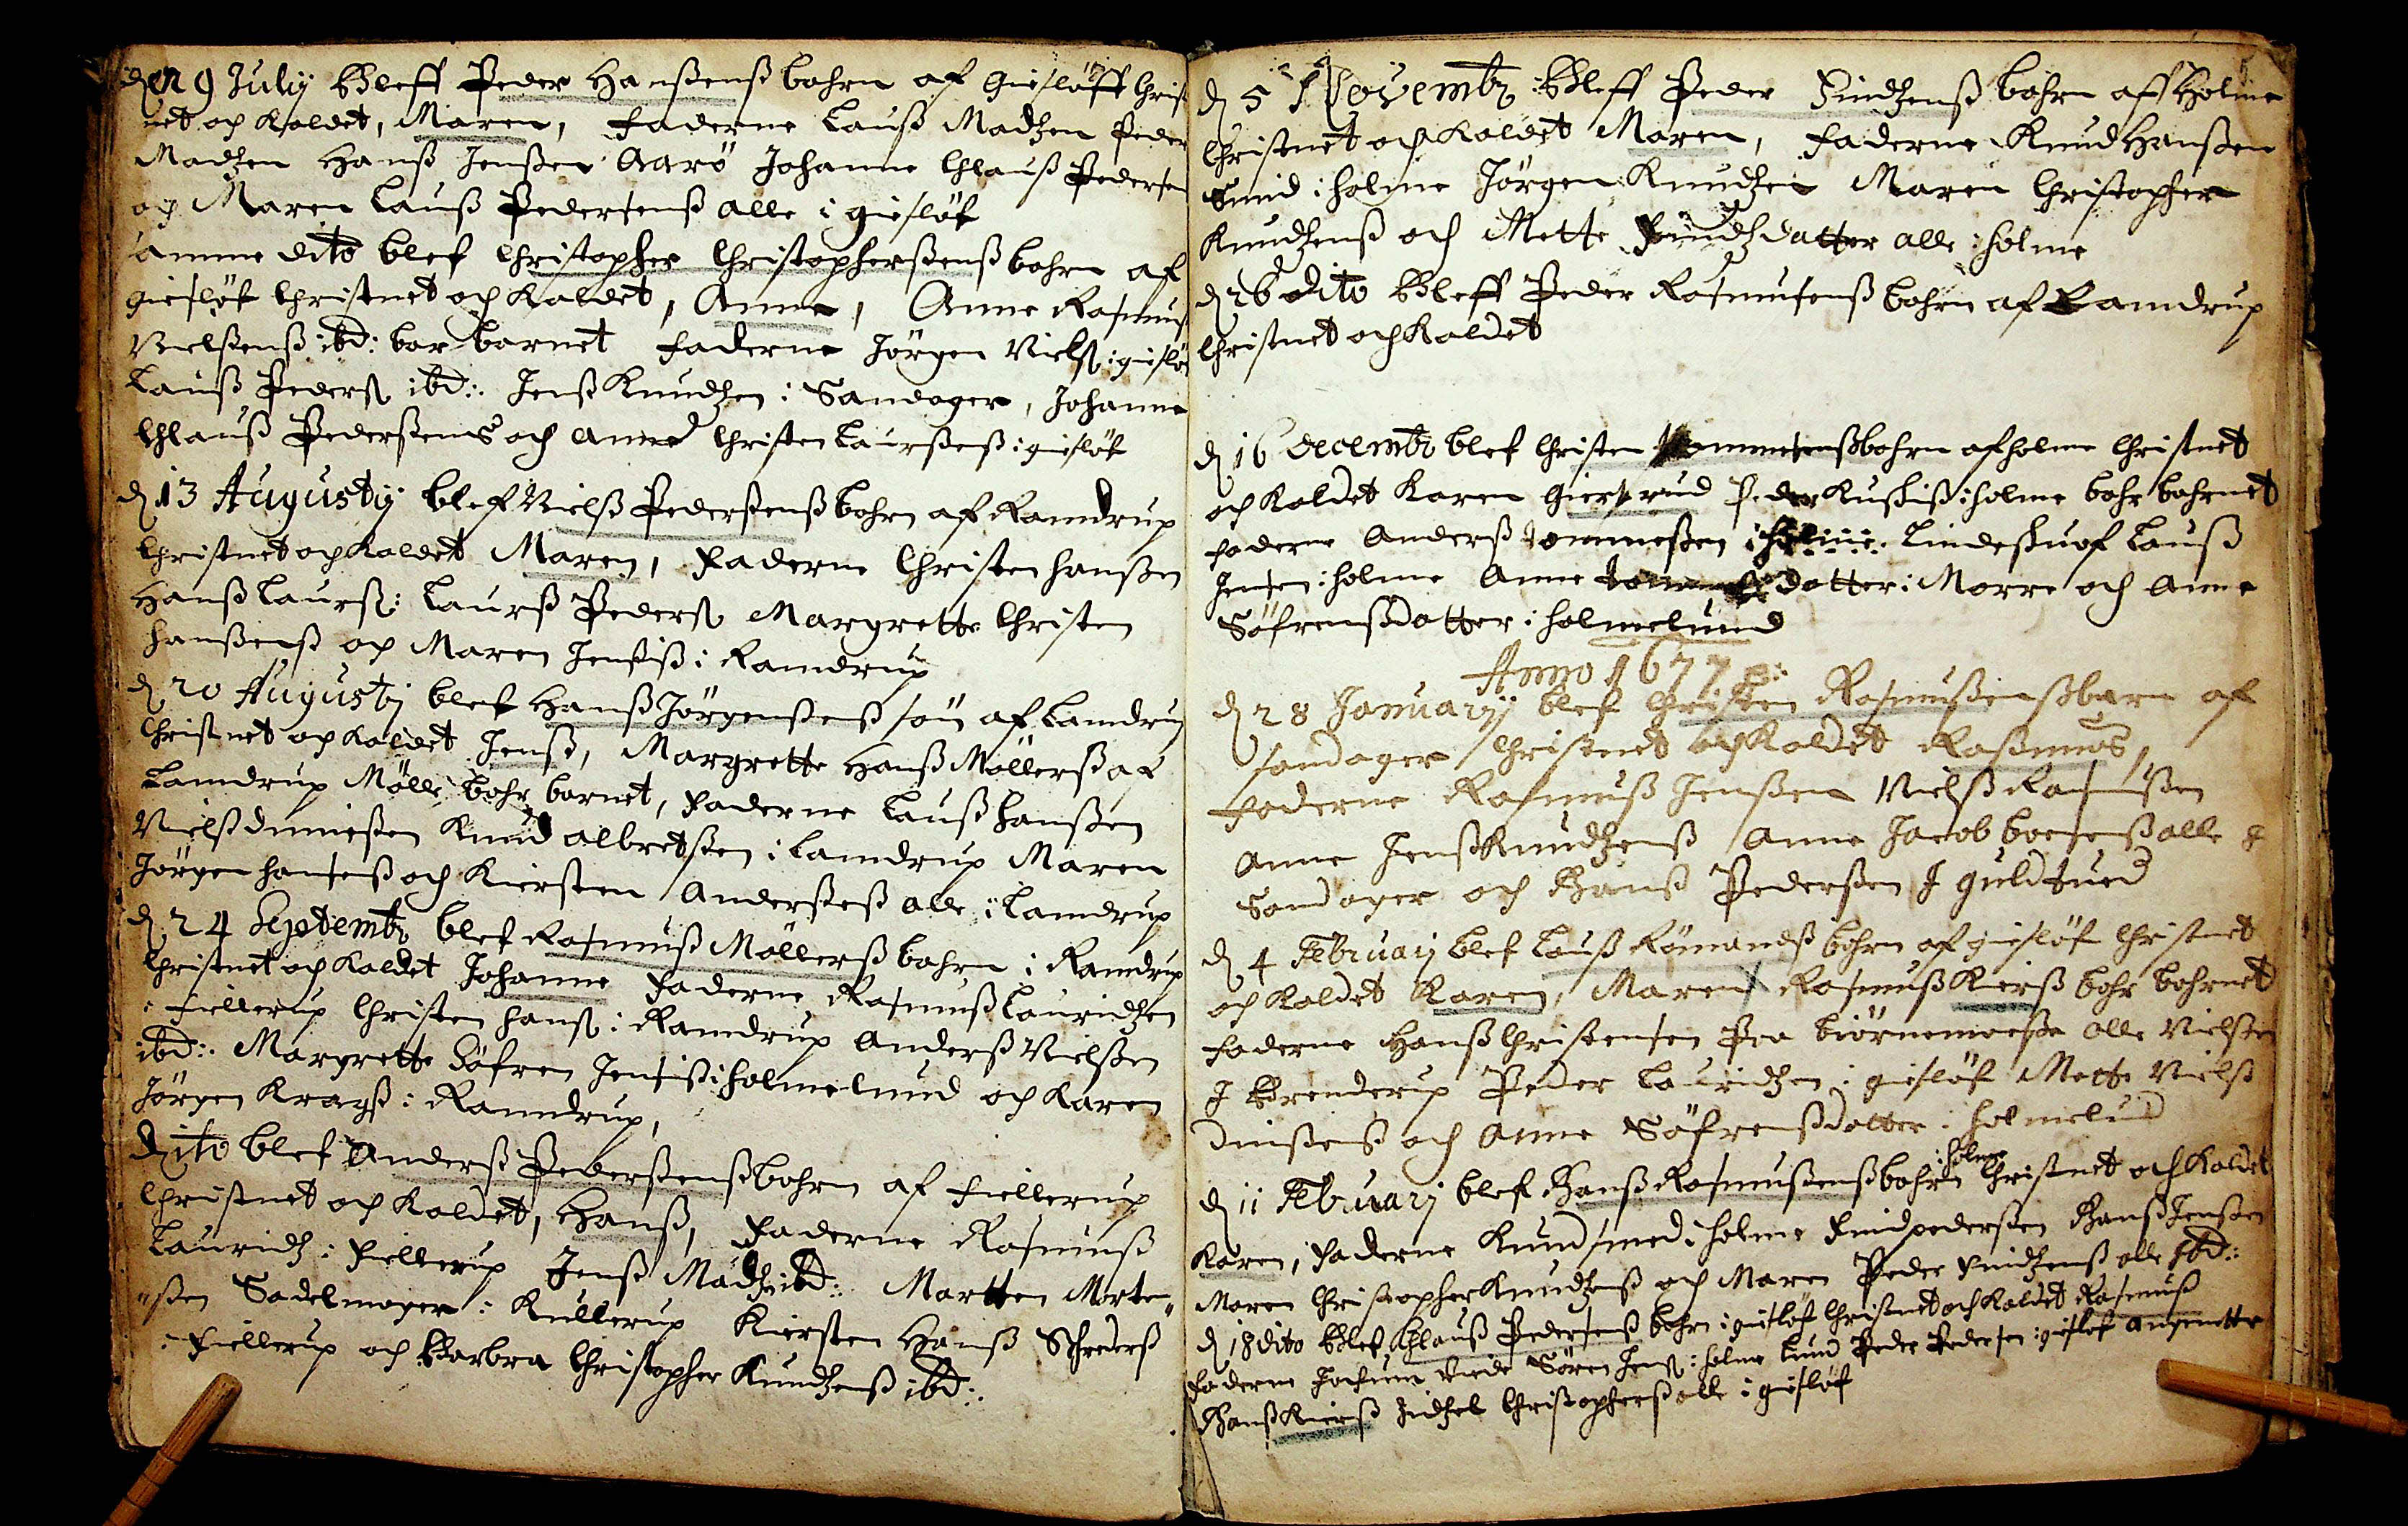den 9 Julij Bleff Peder Hanssenss Bahrn af Giesløff Christ
net och kaldet, Maren, Faderne Lauss Madtzen Peder
Madtzen Hanss Jenssen Aarø Johanne Chlaus Pedersen
och Maren Lauss Pedersensa alle i giesløf
samme dito blef Christopher Christopherssenss bahrn af
giesløf christnet och kaldet, Anne, Anne Rasmus 
Nielssenss ibd: bar barnet Faderne Jørgen Niels i gieslø
Lauss Peders ibd:. Jens Knudtzen i Sandager, Johanne
Chlaus Pederssens och Anne Christen Laurssenss i giesløf
d 13 Augustij bleff Nielss Pederssenss bahrn af Ramdrup
Christnet och kaldet Maren, Faderne Christen Hanssen
Hanss Laurss: Laurs Pederss Margrette Christen
Henssenss och Maren Jenssiss i Ramdrup
d 20 Augustj blef Hanss Jørgenssenss søn af Lamdrup
christenet och kaldet Jenss, Margrette Hanss Møllerss af
Lamdrup Mølle bahr barnet, faderene Lauss Hanssen
Niels D##iessen Knud albretssen i Lamdrup Maren
Jørgen Hansenss och Kiersten Andersens alle i Lardrup
d 24 Septembr blef Rasmuss Møllerss bahrn i Rarndrup
christnet och kaldet Johanne Faderne Rasmuss Lauridtzen
i Fiellerup Christen Hanss i Ramdrup Anderss Nielssen
ibd:. Margrette Søfren Jensiss i Holmelund och Karen
Jørgen Kragss i Ramdrup,
dito blef Anderss Pederssenss Bahrn af Fiellerup
christnet och kaldet, Hanss, Faderene Rasmuss
Lauridtz i Fiellerup Jenss Madtzen ibd:. Morten Morten¬
ssen Sadelmoger i Kullerup Kiersten Hanss Schrederss
i Fiellerup och Barbra Christopher Knudtzens ibd:.
5.
d 5 7 ##embr Bleff Peder ##Findtzenss bahrn aff Holme
christnet och Kaldet Maren, Faderne Knud Hanssen
Smid i Holme Jørgen Knudtzes Maren Christopher
Knudtzens och Mette ##Findtzdatter alle i Holme
d 26 dito Bleff Peder Rasmusenss bahrn af Ramdrup
christnet och kaldet
d 16 decembr blef Christen ##tommesenss bahrn af holme christnet
och kaldet Karen Giertrud Peder Kuskis## i holme bahr bahrnet
Fadere Anderss tommesen i Holme Lindeskuof Lauss
Jensen i holme Anne tommesdatter i Morre och Anne
Søfrenssdatter i Holmelund
Anno 1677
d 28 Januarij blef Christien Rasmussenss barn af
sandager christned och kaldet Rassmus,
faderne Rasmuss Jenssen Nielss Rasmussen
Anne Jens Knudtzenss Anne Jacob boesess alle I
Sandager och Hanss Pederssen I Guldtue##
d 4 Februarj blef Lauss Rønnendss## bahrn af giesløf christnet
och kaldet Karen, Maren Rasmuss Kierss bahr bahrnet
Faderne Hanss Christensen P## biørnemoste## Olle Nielssen
I Brenderup, Peder Lauridtzen i giesløf Mette Nielss
dinssenss## och Anne Søfrenssdatter i Holmelund
i Holme
d 11 Februuarj blef Hanss Rasmussenss bahrn christnet och kaldet
Karen, Faderne Knud smed i Holme Find pederssen Hanss Jenssen
Maren Christopher Knudtzens och Maren Peder Findtzens alle ibd.:
D 18 dito blef Chlaus Pedersenss bahrn i giesløf christenet och kaldet Rasmuss
Faderne Jochum viede## Søren Jenss i holme Lund Peder Pedersen i giesløf angnette
Hans Kierss Zidtzel Christopherss alle i giesløf
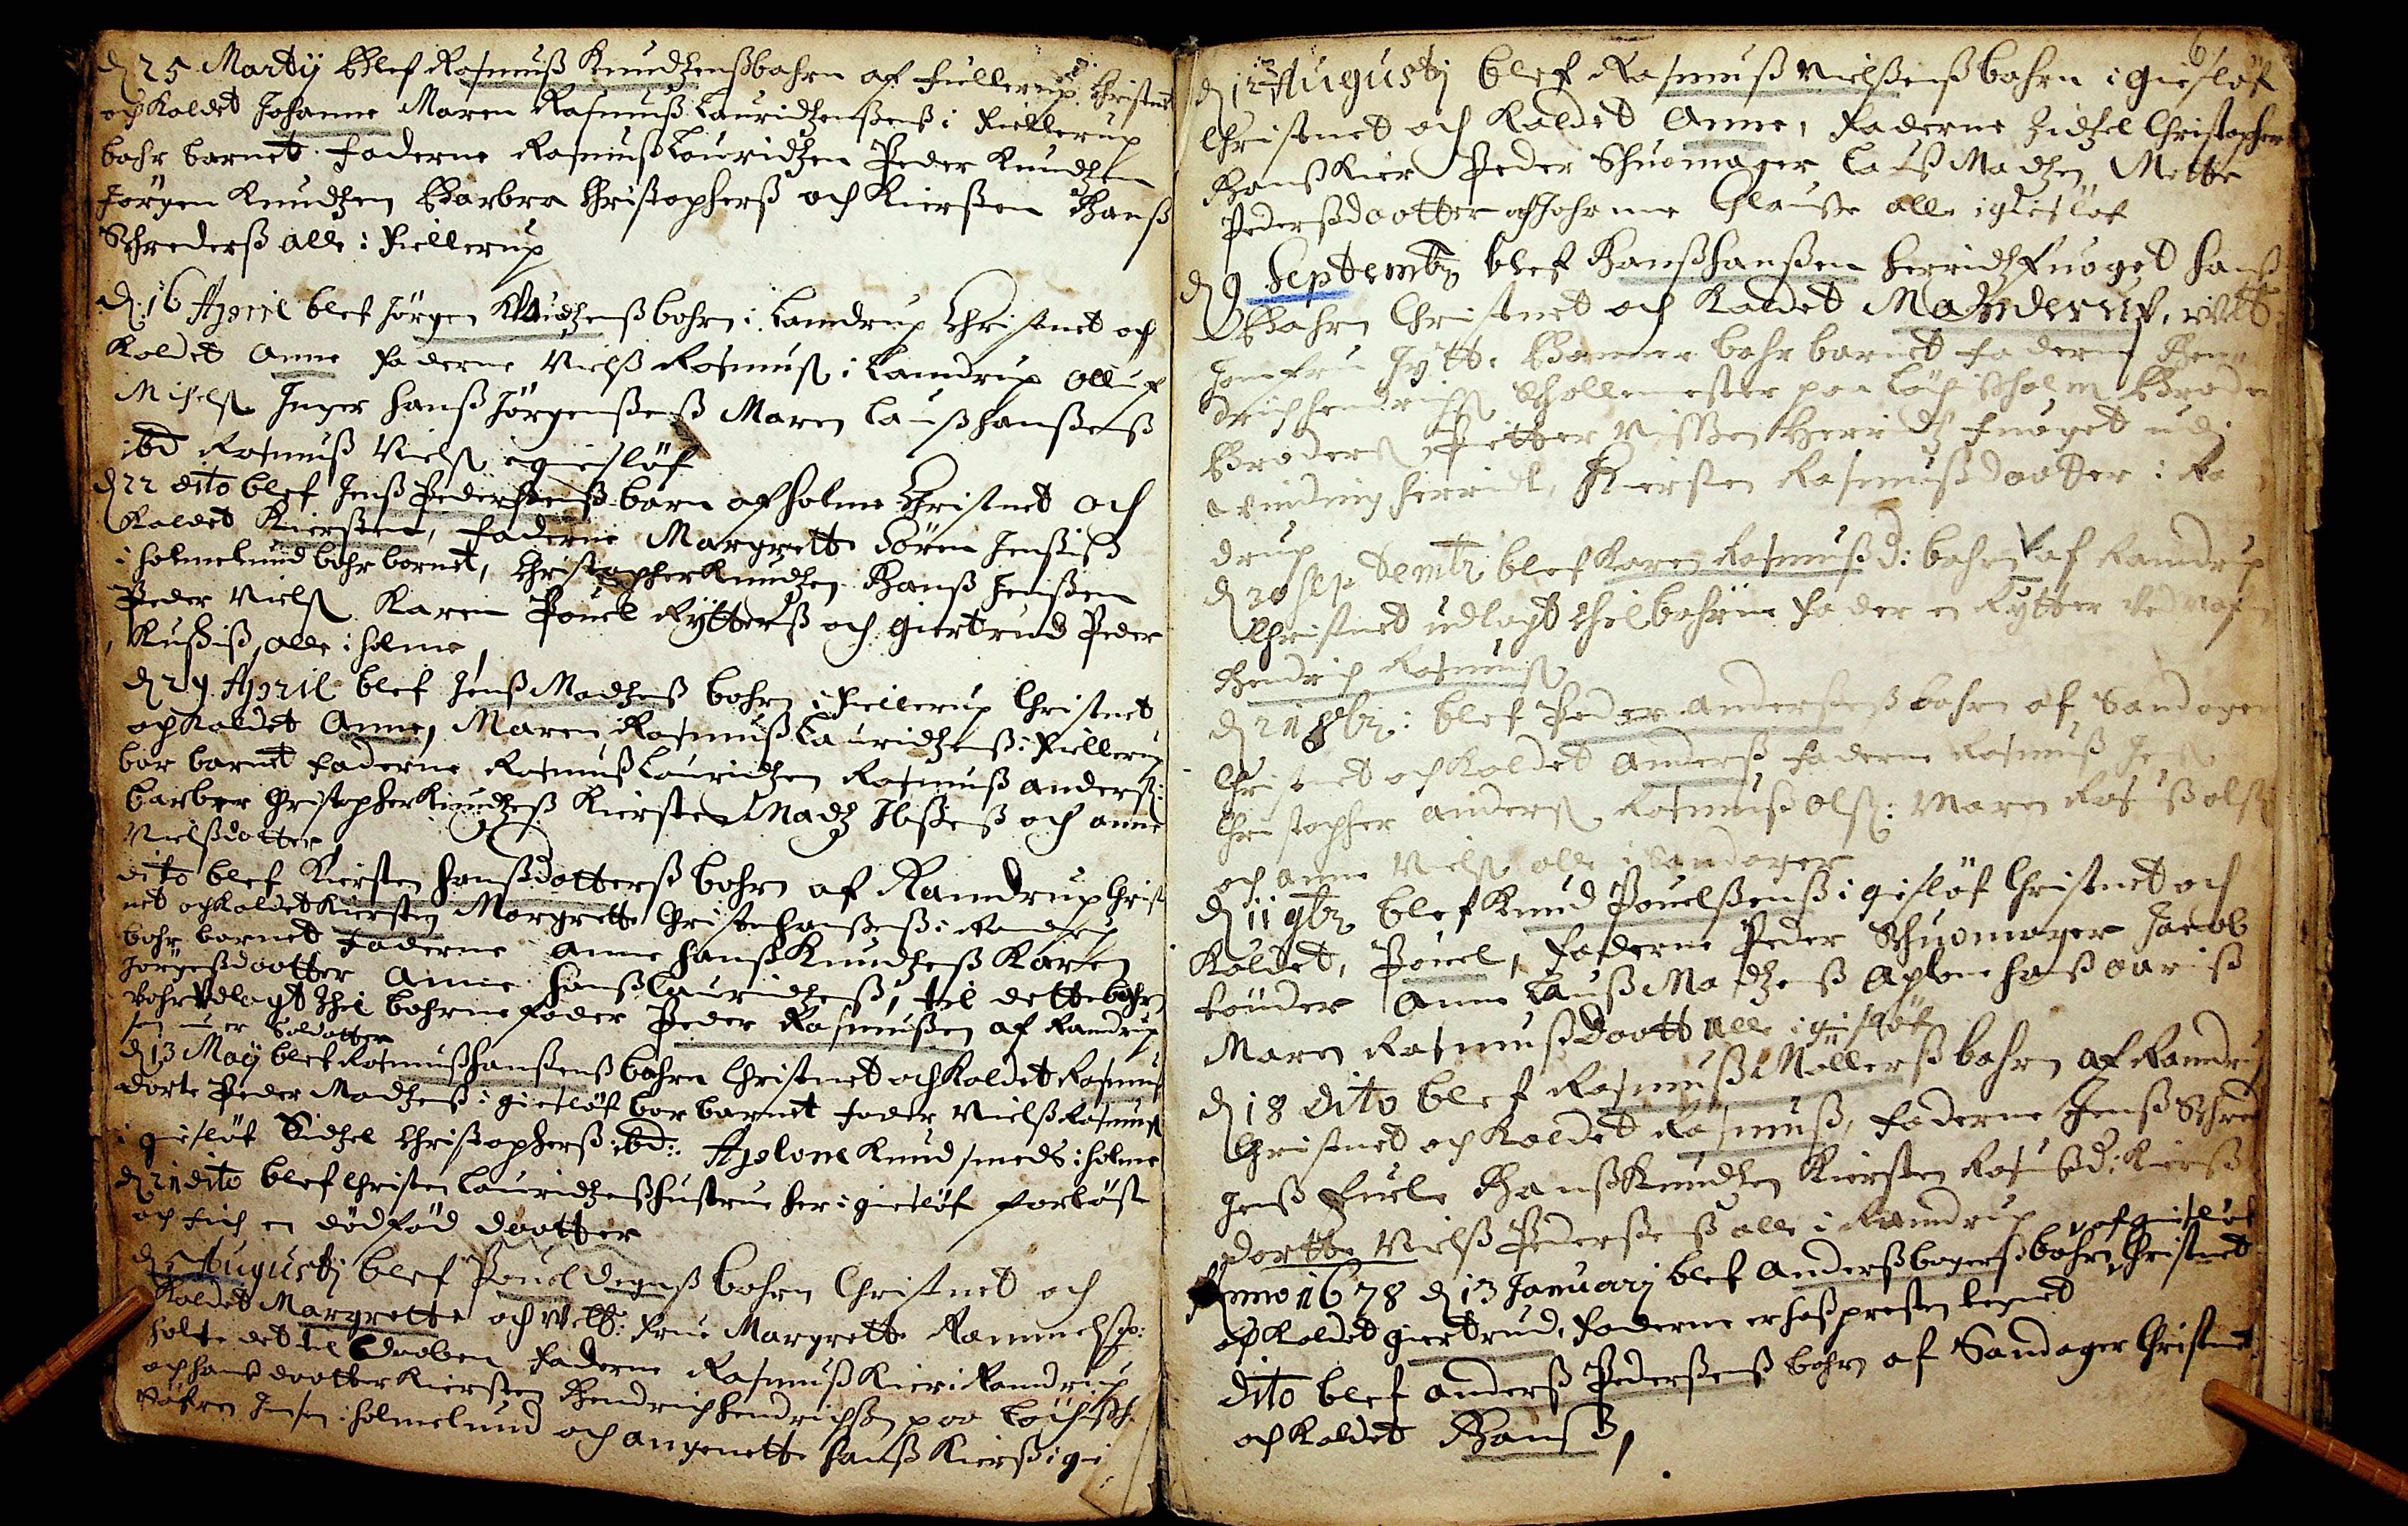D 25 Martij Bleff Rasmuss Knudtzenss bahrn af Fiellernup Christenet
och kaldet Johanne Maren Rasmuss Lauridtzenssenss i Fiellerup
bahr barnet. Faderne Rasmuss Lauridtzen Peder Knudtzen
Jørgen Knudtzen, Barbra Christopherss och Kirsten Hanss
Schrederss alle i Fiellerup
D 16 April blef Jørgen Klaudtzenss bahrn i Lamdrup Christnet och
kaldet Anne Faderne Nielss Ramuss i Lamdrup Olluf
Michels Inger Hanss Jørgenssenss Maren Laus Hanssenss
ibd Rasmuss Nielss i giesløf
d 22 dito blef Jenss Pederssenss barn af Holme Christnet och
kaldet Kiersten, Faderne Margrette Søren Jenssiss
i Holmelund bahr barnet, Christopher Knudtzen. Hanss Jenssen
Peder Niels Karen Pouel Rytterss och Giertrud Peder
##Kuskiss, alle i Holme
d 27 Apriil blef Jenss Madtzenss bahrn i Fiellerup Christnet
och kaldet Anne, Maren Rasmuss Lauridtzenss i Fiellerup
bar barnet Faderne Rasmuss Laouridtzen Rasmuss Anderss
Barbra Christopher Knudtzenss Kiersten Madtz Ibssenss och Anne
Nielssdatter
dito blef Kiersten Hanssdatterss Bahrn af Ramdrup Chri
net og kaldet Kiersten Margrette Christen Hanssenss i Randrup
bahr barnet Faderne Anne Hanss Knudtzenss Karen
Jørgenssdaatter Anne Hanss Lauridtzenss, til dette bahrn
vahr vdlagt thil bahrnefader Peder Rasmussen af Ramdrup
ssom nu er Soldatter
d 13 Maij blef Rasmuss Hanssenss bahrn Christnet och kaldet Rasmuss
Dorte Peder Madtzens i Giesløf bar barnet. fader Niels Rasmuss
i Giesløf Sidtzel Christopherss ibd:, Apelone Knud smeds i Holme
d 24 dito blef Christen Lauridtzenss Hustrue her i Giesløf forløst
och fich en dødfød daatter
d 5 Augustij blef Pouel Degnss bahrn Christnet och
kaldet Margrette og Well: Frue Margrette Ræ##chss:
holte det til Daaben Faderne Rasmuss Kier i Ramdrup
och hans daatter Kiersten Hendrich Hendrichss paa Løchissh.
Søfren Jensen i Holmelund och Angenette Hanss Kierss i gi  
6
d 12 Augustij blef Rasmuss Nielssenss bahrn i Giesløf
christnet och kaldet Anne, Faderne, Zidtzel Christopffer
Hanss Kier Peder Shuemager ##ats Madtzen, Mette
Pederssdaatter och Johanne Chlaus## alle i giesløf
d 9 Septembr blef Hanss Hanssen Herridtzfuoget Hanss
Bahrn Christnet och kaldet Ma##derux##, vellb:
Jomfrue J##tte ## bahr barnet Faderne Hen¬
drich hendricha Schollemester paa Løchisholm Broder
Broders Petter Nissen Herridtzfuoget udi
##vinding herridt Kiersten Rasmussdattter i Ra
drup
d 30 Septembr blef Karen Rasmussd: bahrn af Ramdrup
Christnet udlagt thil bahrne Fader en Rytter ved Nafn
Hendrich Rasmuss
d 21 8br: blef Peder Anderssenss bahrnaf Sandager
christnet och kaldet Anderss Faderne Rasmuss Jenss
Christopher Anders Rasmuss Olss: Maren Rasmuss olss
och Anne Nielss alle i Sandager
d 11 9br. bleff Knud Pouelssenss i Gisløf Christnet och
kaldet, Pouel, Faderne Peder Schuomager Jacob
tønder Anne Lauss Madtzenss Aplone Hoss##riss
Maren Rasmuss D##tt alle i gisløf
d 18 dito blef Rasmuss Møllerss bahrn af Ramdrup
christned och kaldet Rasmuss, Faderne Jenss Schred 
Jenss Fuel Hanss Kbudtzen Kiersten Rasmusd: Kierst 
Dortte Nielss Pederssenss alle i Ramdrup
v af Giesløf
Anno 1678 d 13 Januarij blef Anderss bagerss bahrn christnet
och kaldet Giertrud, Foderne er hasspresten Legnet
dito blef Anderss Pederssenss bahrn af Sandager Christnet
och kaldet Hanss,